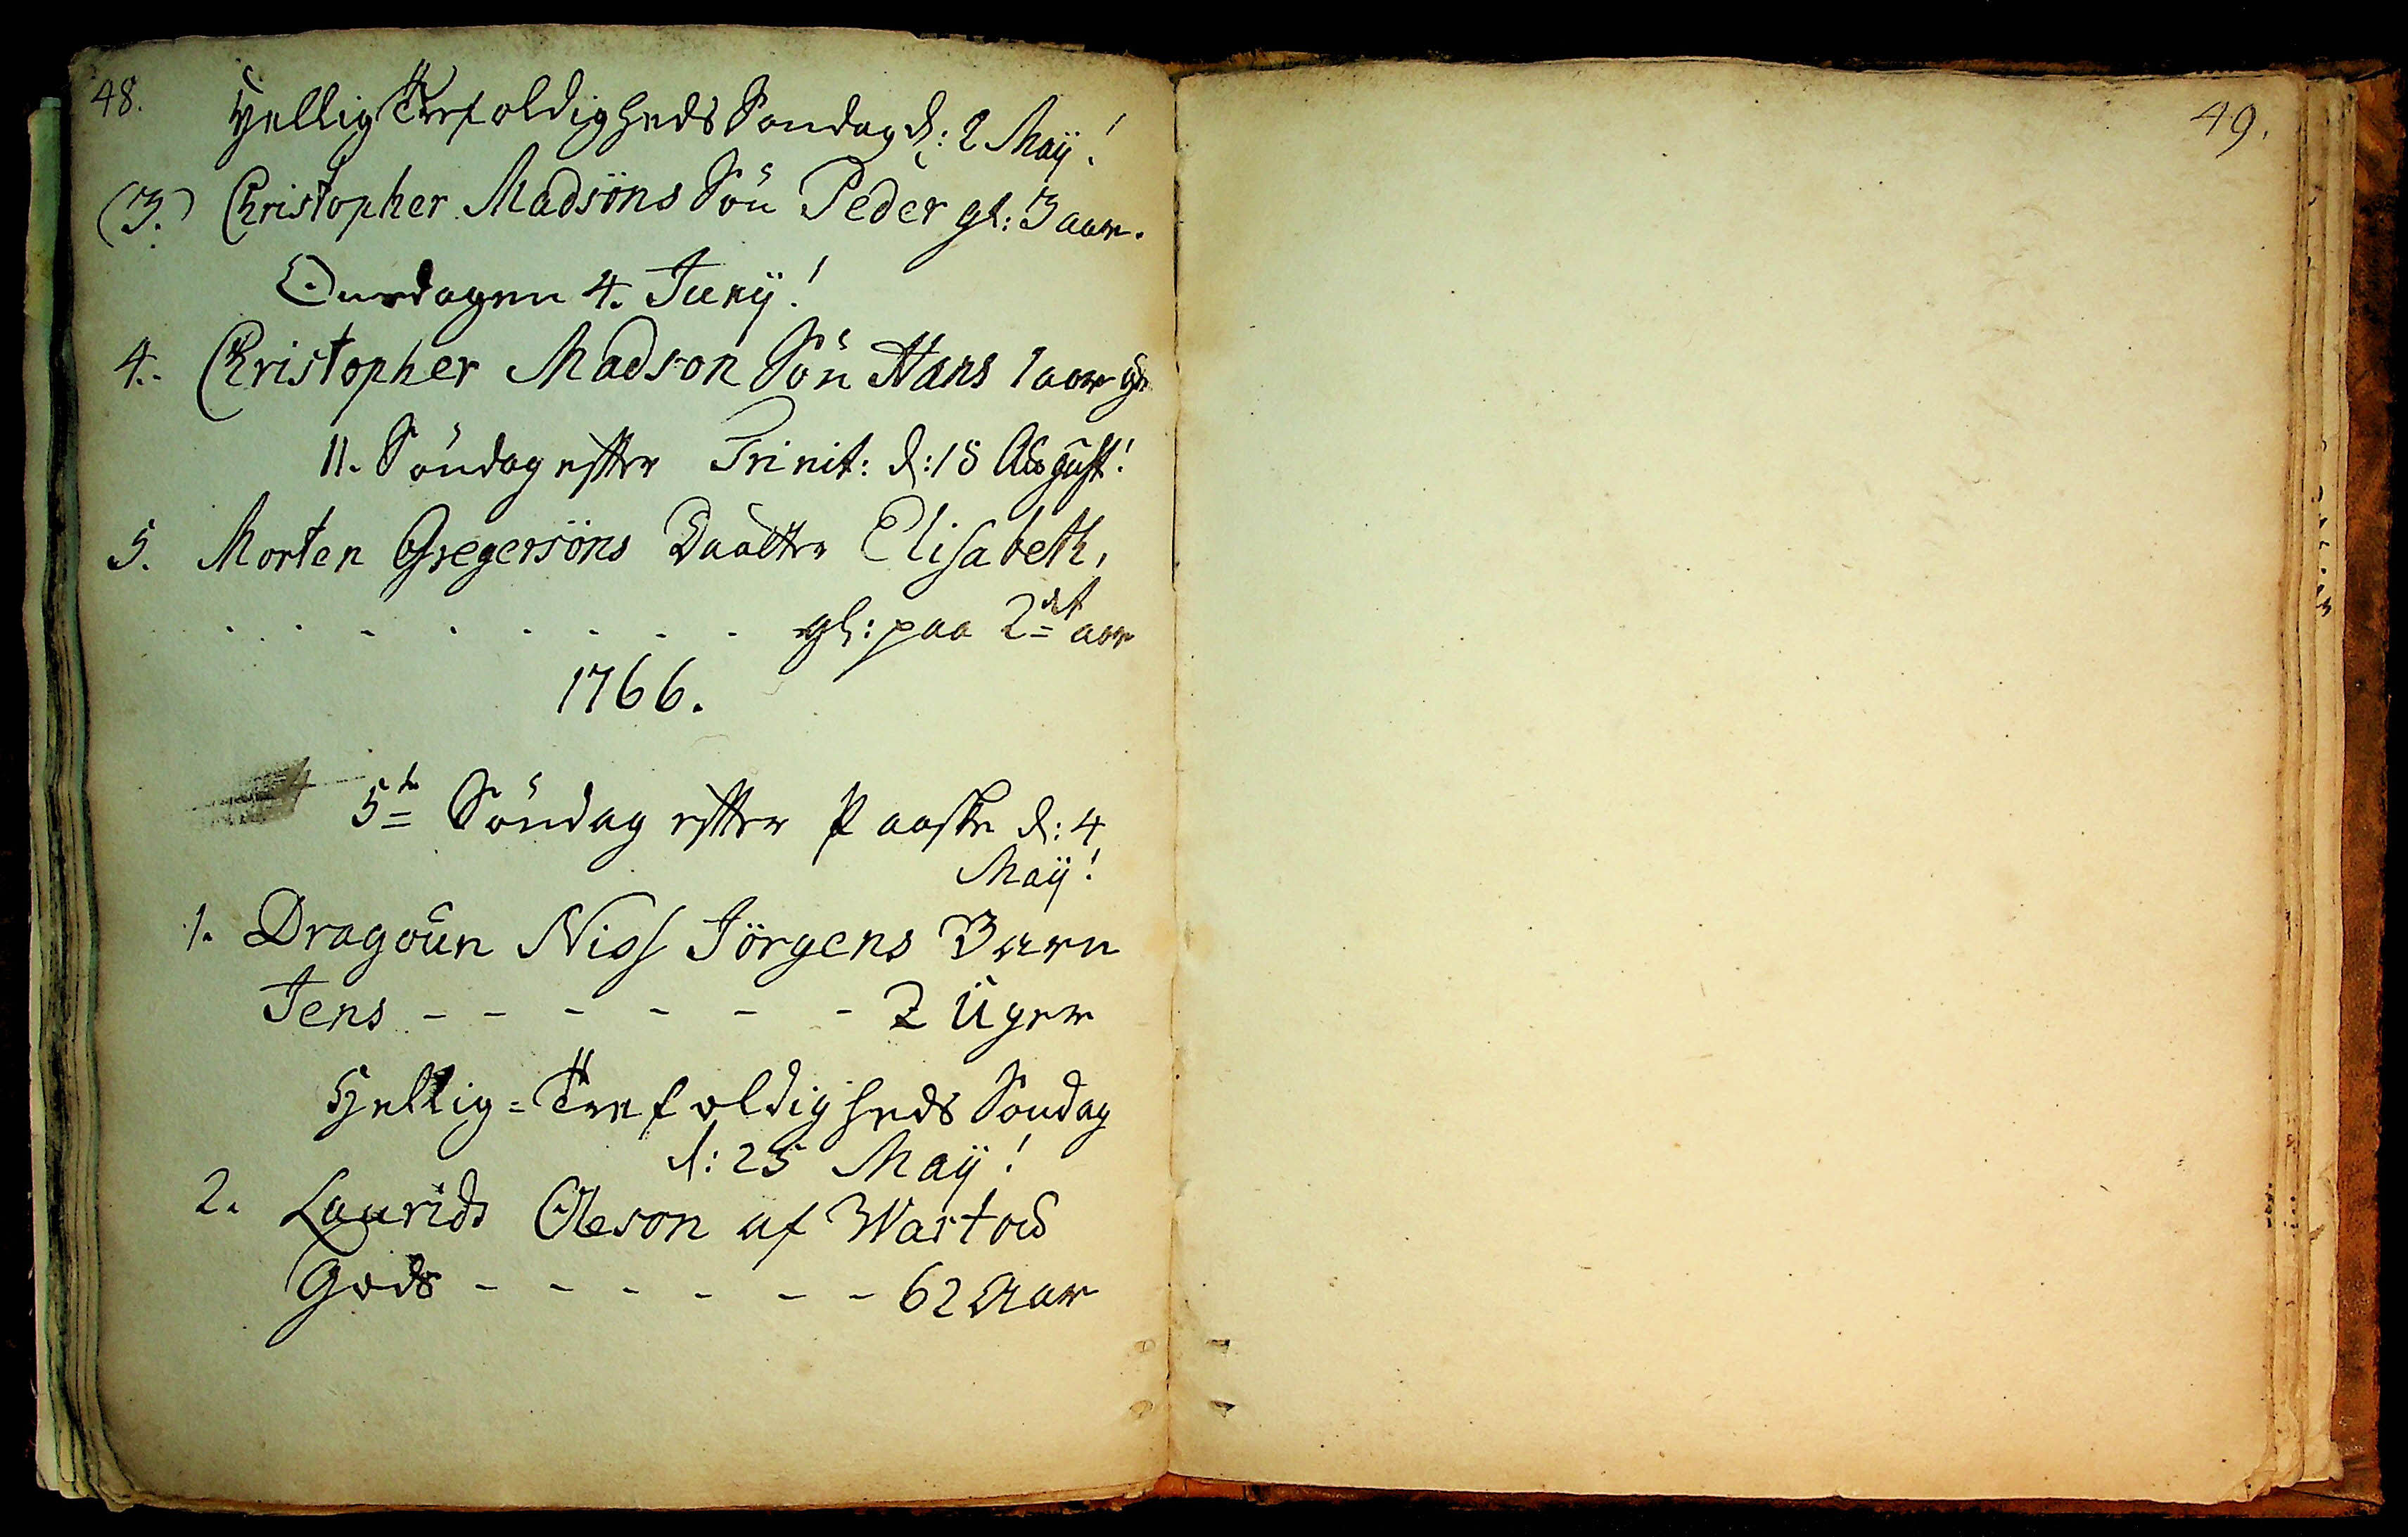48.
Hellig Trefoldigheds Søndag d: 2 Maij!
(3.) Christopher Madsøns Søn Peder gl: 3 aar.
Onsdagen 4. Junij!
4. Christopher Madsøn Søn Hans 1 Aar gl
11. Søndag efter Trinit: d: 15 August!
5. Morten Gregersøns Daatter Elisabeth.
 gl: paa 2det Aar
1766.
5te Søndag efter Paaske d: 4
Maij!
1. Dragoun Niss Jørgens Barn
Jens 
2 Uger
Hellig = Trefoldigheds Søndag
d: 25 Maij!
2. Laurids Olesøn af Wartou
Gods
62 Aar
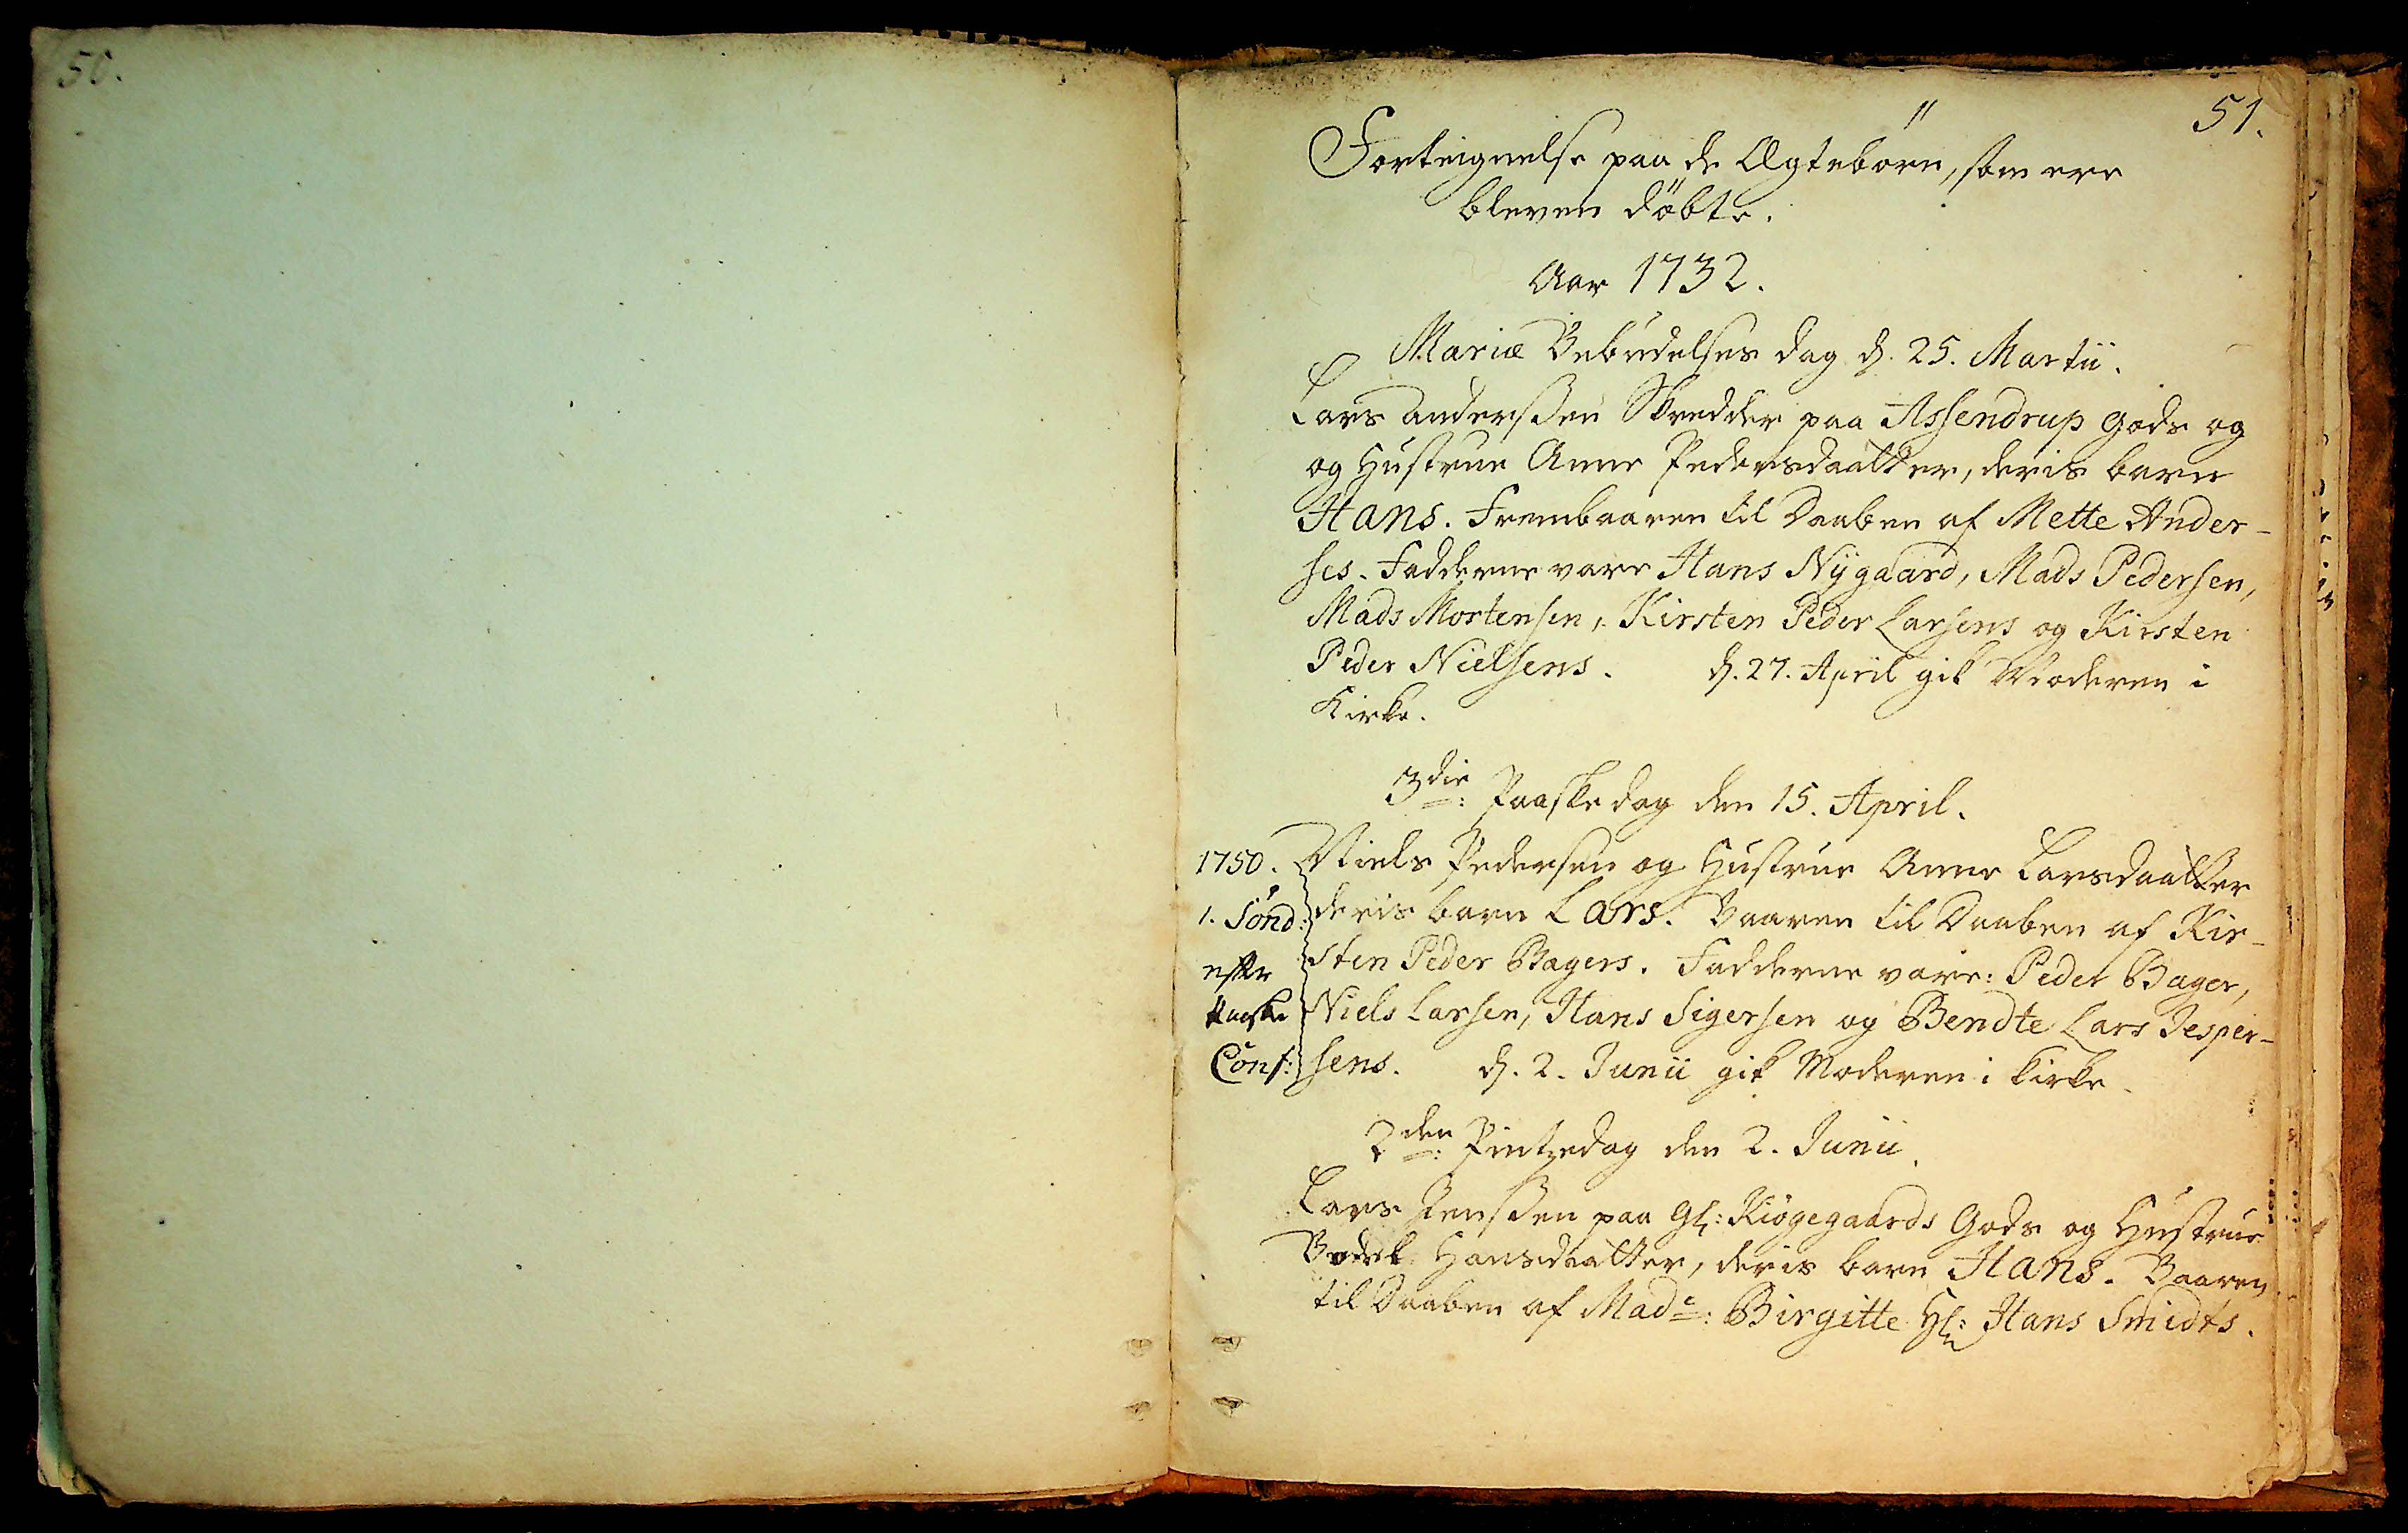51. 
Forteignelse paa de Ægtebørn, som ere
bleven døbte.
Aar 1732.
Mariæ Bebudelses dag d. 25. Martii.
Lars Andersen Skredder paa Assendrup gods og
og Hustrue Anne Pedersdaatter, deris barn
Hans. Frembaaren til Daaben af Mette Ander¬
ses. Fadderne vare Hans Nygaard, Mads Pedersen,
Mads Mortensen, Kirsten Peder Larsens og Kirsten
Peder Nielsens. d. 27. April gik Moderen i
Kirke.
3die: Paaskedag den 15. April.
1750 
1.Sønd:
efter 
Paaske
Conf: 
Niels Pedersen og Hustrue Anne Larsdaatter
deris barn Lars. Baaren til Daaben af Kir¬
sten Peder Bagers. Fadderne vare: Peder Bager,
Niels Larsen, Hans Sigersen og Bendte Lars Jesper
sens. d. 2. Junii gik Moderen i Kirke. 
2den: Pintzedag den 2. Junii.
Lars Jensen paa Gl: Kiøgegaards Gods og Hustrue
Bodil Hansdaatter, deris barn Hans. Baaren
til Daaben af Made Birgitte Hr: Hans Smidts.